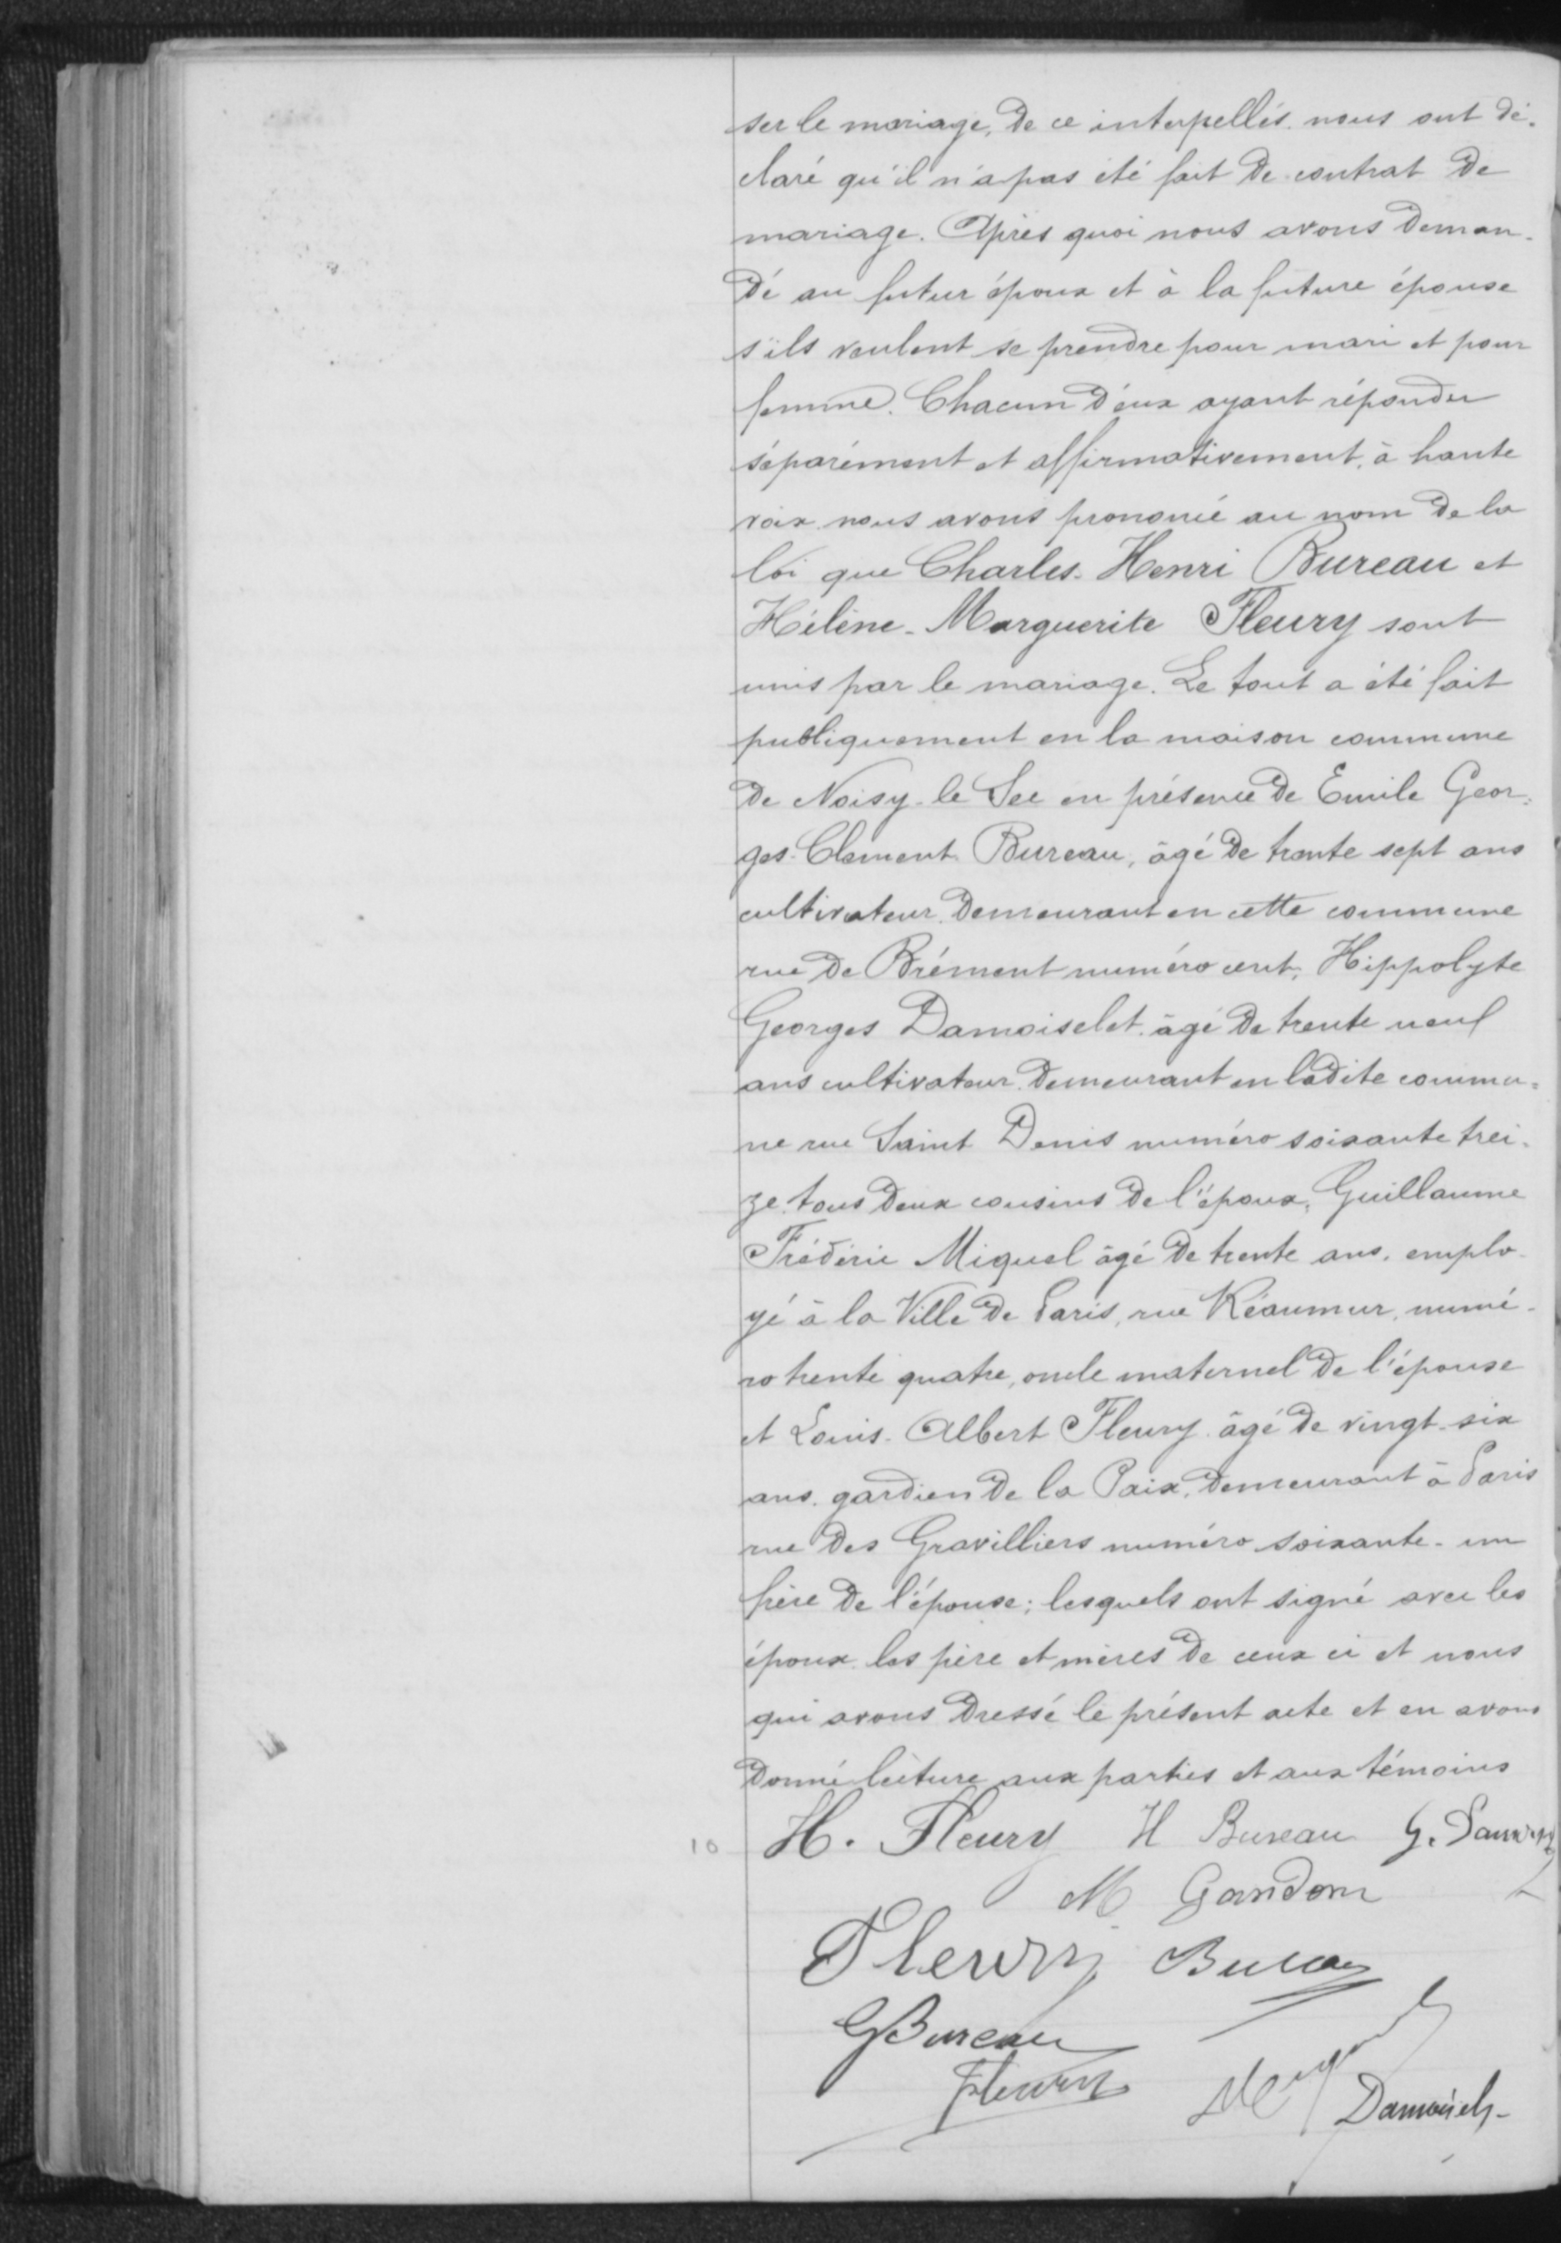sur le mariage, de ce interpellés nous ont dé-
claré qu'il n'apas été fait de contrat de
mariage. Après quoi nous avons deman-
dé au futur époux et à la future épouse
s'ils veulent se prendre pour mari et pour
femme. Chacun d'eux ayant répondu
séparément et affirmativement à haute
voix nous avons prononcé au nom de la
loi que Charles -Henri Bureau et
Hélène-Marguerite Fleury sont
unis par le mariage. Le tout a été fait
publiquement en la maison commune
de Noisy-le-Sec en présence de Emile Geor
ges-Clement Bureau, âgé de trente sept ans
cultivateur. Demeurant en cette commune
rue de Brémont numéro cent, Hippolyte
Georges Damoiselet, âgé de trente neuf
ans cultivateur. demeurant en ladite commu-
ne rue Saint Denis numéro soixante trei-
ze tous deux cousine del'époux Guillaume
Frédéric Miquel âgé de trente ans, emplo-
yé à la Ville de Paris, rue Réaumur, numé-
ro trente quatre, oncle maternel de l'épouse
et Louis Albert Fleury. âgé de vingt-six
ans. gardien de la Paix, demeurant à Paris
rue des Gravilliers numéro soixante-un
frère de l'épouse; lesquels ont signé avec les
époux les père et mères de ceux ci et nous
qui avons dressé le présent acte et en avons
donné lecture aux parties et aux témoins
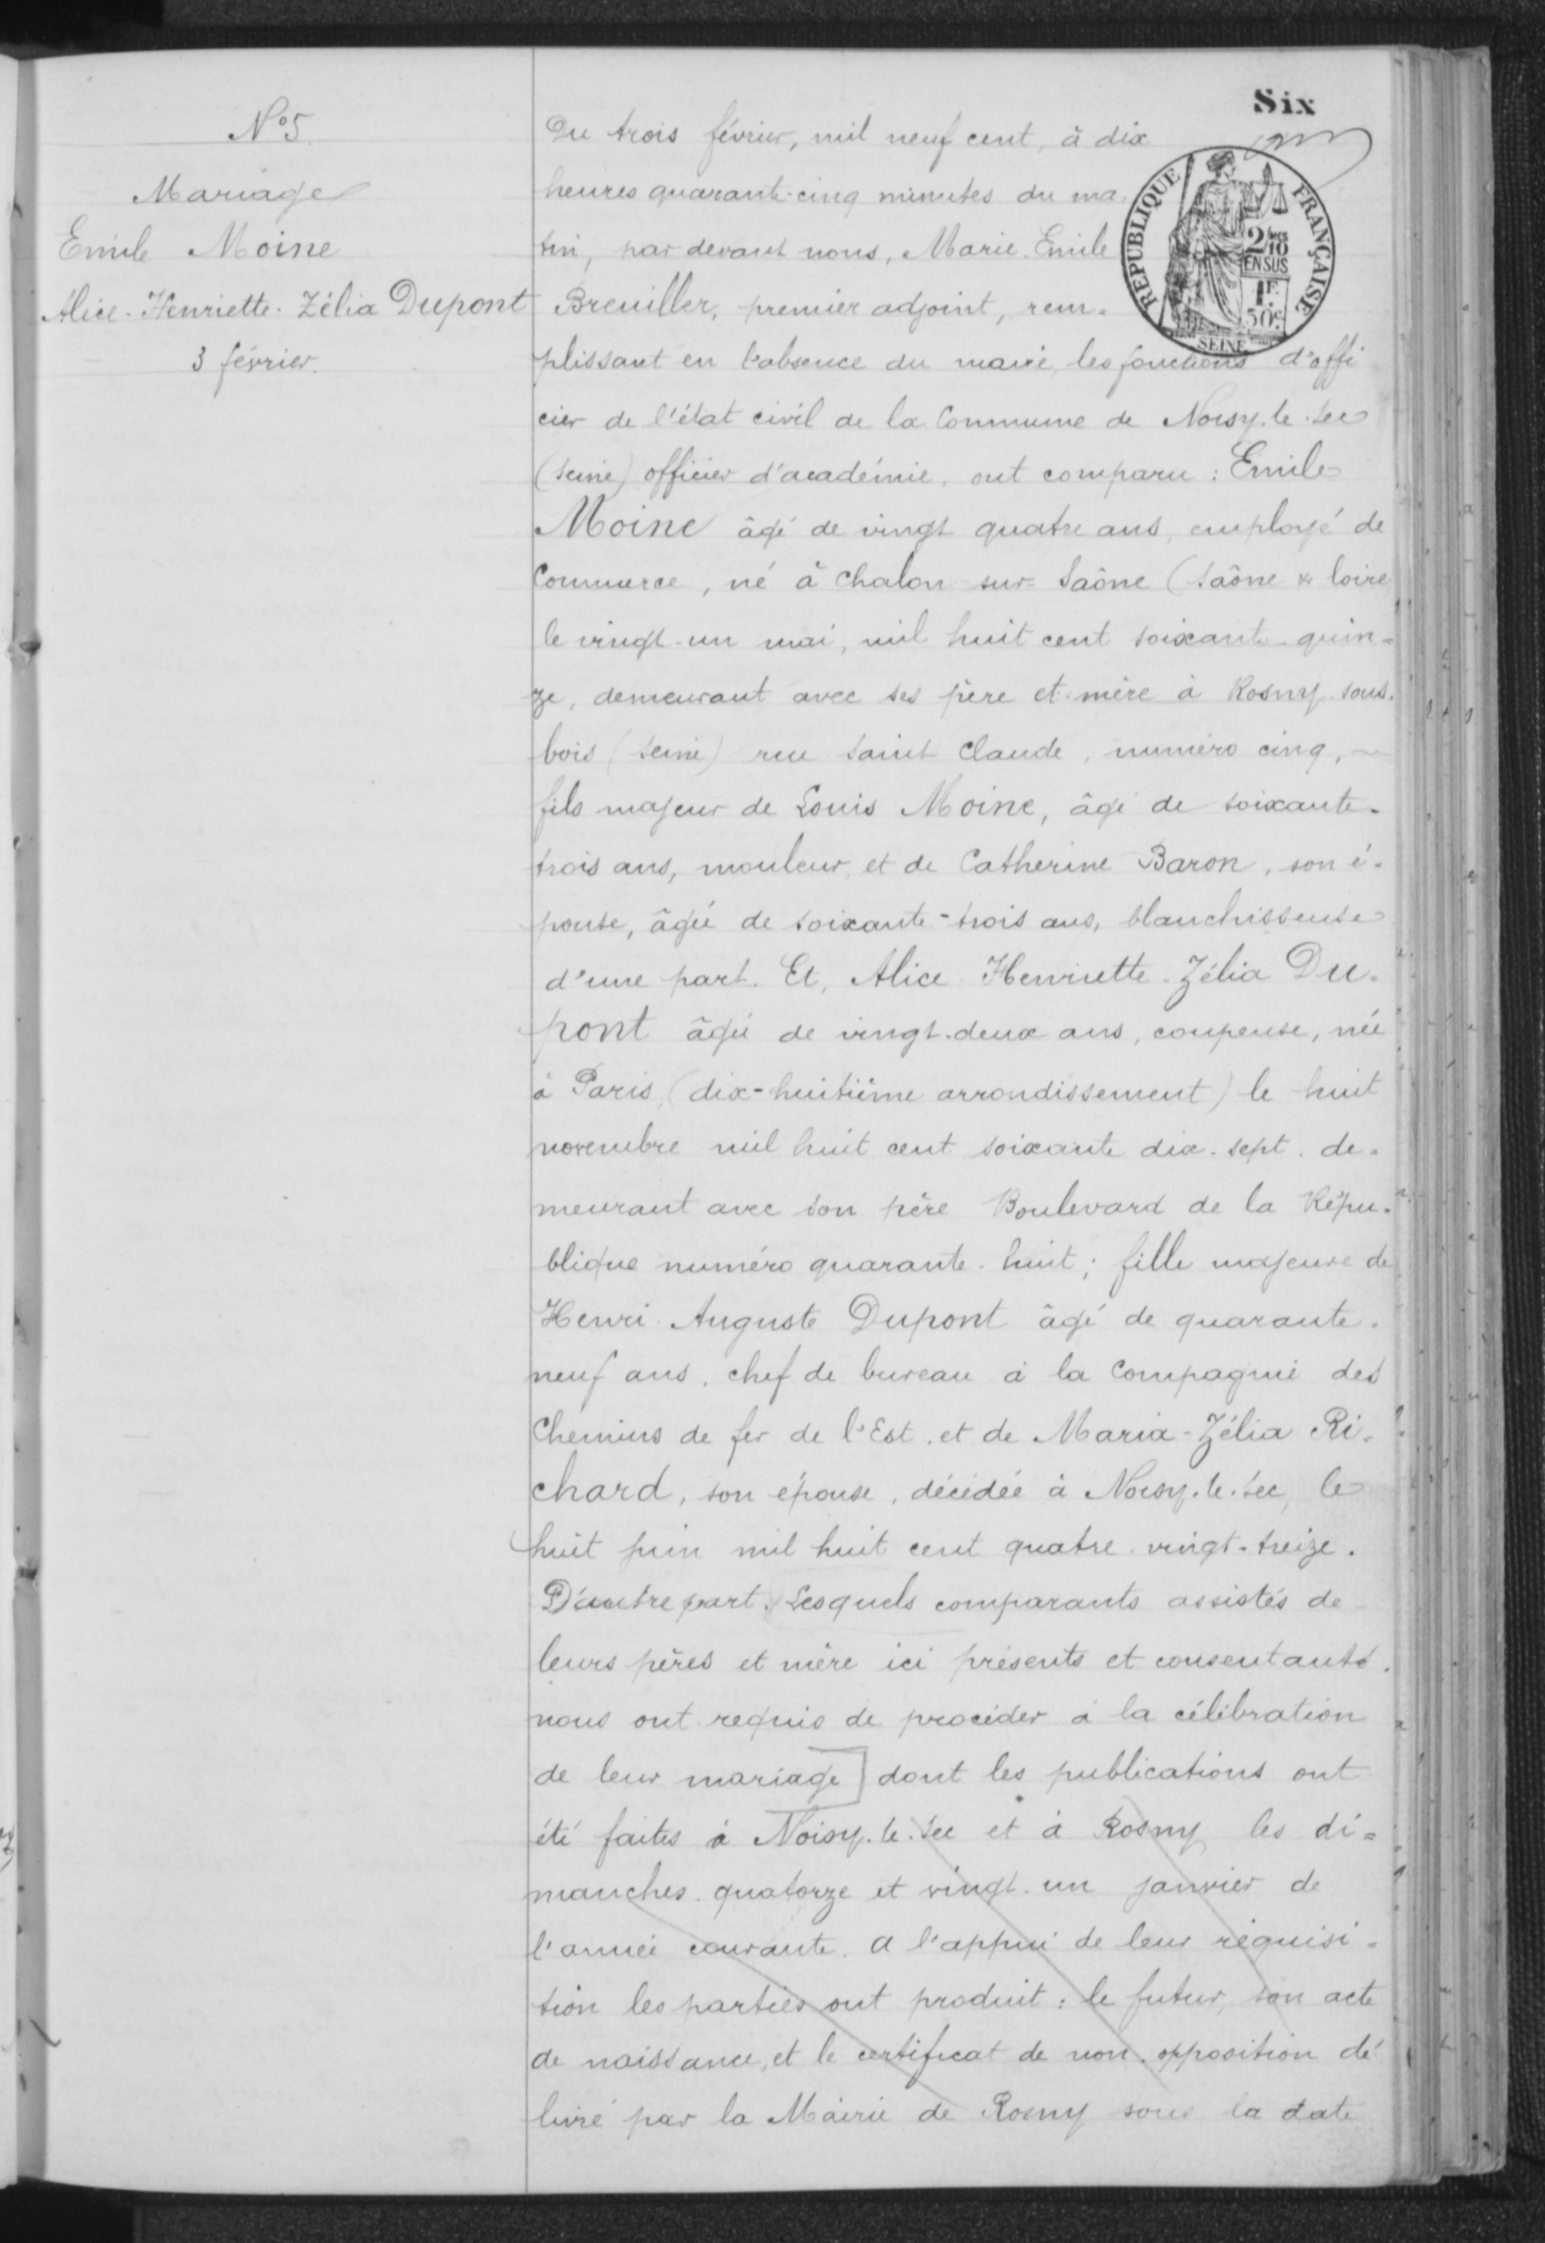N°5
Mariage
Emile Moine
Alice-Henriette-Zélia Dupont
3 février
Du trois février, mil neuf cent, à dix
heures quarante-cinq minutes du ma
tin, par devant nous, Marie-Emile
Breuiller, premier adjoint, rem-
plissant en l'absence du mari, les fonctions d'offi
cier de l'état civil de la Commune de Noisy-le-Sec
(Seine) officier d'académie, ont comparu: Emile
Moine âgé de vingt quatre ans, employé de
Commerce, né à Chalon-sur-Saône (Saône et Loire)
le vingt-un mai, mil huit cent soixante-quin
ze, demeurant avec ses père et mère à Rosny-sous
bois (Seine) rue Saint Claude, numéro cinq, -
fils majeur de Louis Moine, âgé de soixante-
trois ans, mouleur et de Catherine Baron, son é-
pouse, âgée de soixante-trois ans, blanchisseuses
d'une part. Et Alice Henriette-Zélia Du-
pont âgée de vingt-deux ans, coupeuse, née
à Paris (dix-huitième arrondissement) le huit
novembre mil huit cent soixante dix-sept de-
meurant avec son père Boulevard de la Répu
blique numéro quarante-huit; fille majeure de
Henri Auguste Dupont âgé de quarante-
neuf ans. chef de bureau à la compagnie des
Chemins de fer de l'Est, et de Maria-Zélia Ri-
chard, son épouse, décédée à Noisy-le-Sec, le
huit juin mil huit cent quatre-vingt-treize.
D'autre part. Lesquels comparants assistés de
leurs pères et mère ici présents et consentants
nous ont requis de procéder à la célébration
de leur mariage dont les publications ont
été faite à Noisy-le-Sec et à Rosny les di-
manches quatorze et vingt-un janvier de
l'année courante. A l'appui de leur réquisi-
tion les parties ont produit: le futur son acte
de naissance, et le certificat de non-opposition dé
Livré par la Maire de Rosny sous la date